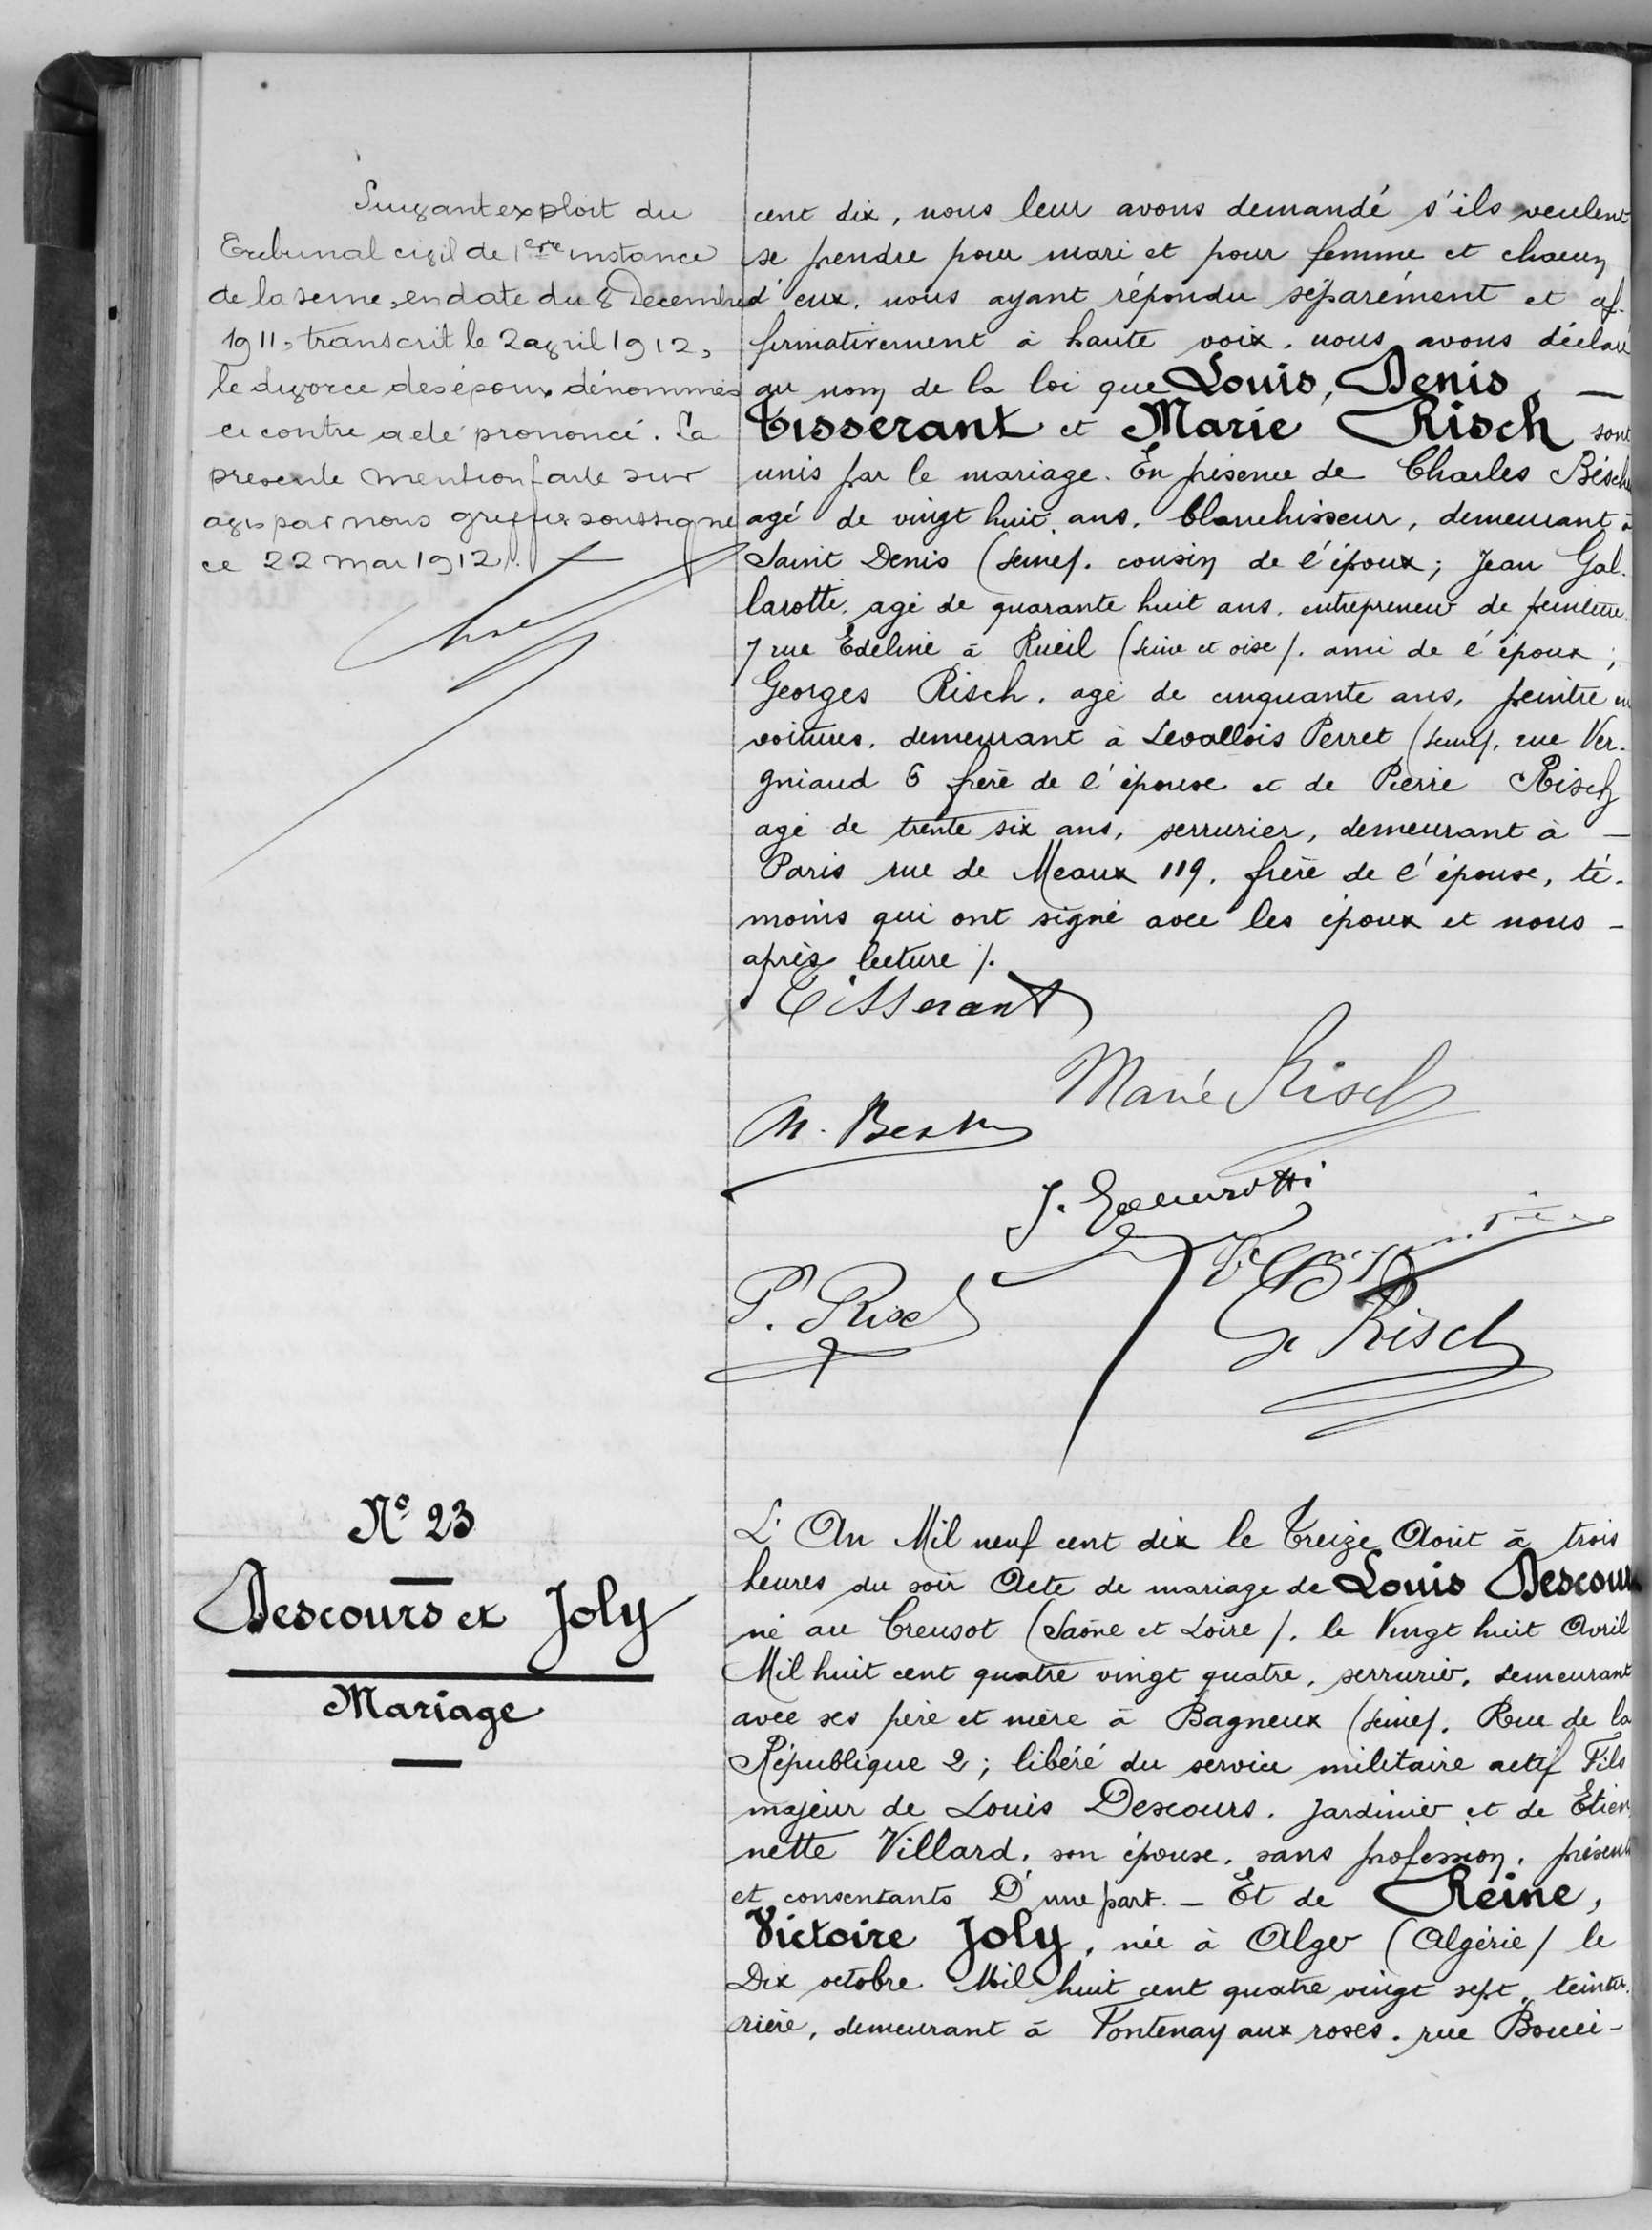cent dix, nous leur avons demandé s'ils veulent
se prendre pour mari et pour femme et chacun
d'eux. nous ayant répondu séparément et af-
firmativement à haute voix. nous avons déclaré
au nom de la loi que Louis, Denis -
Tisserant et Marie Risch sont
unis par le mariage. En présence de Charles Bésch
agé de vingt huit ans. blanchisseur, demeurant à
Saint Denis (Seine). cousin de l'époux; Jean Gal-
larotte, agé de quarante huit ans. entrepreneur de fermeture
7 rue Edeline à Rueil (Seine et Oise). ami de l'époux;
Georges Risch. agé de cinquante ans, peintre sur
voiture. demeurant à Levallois Perret (Seine). rue Ver-
gniaud 6 frère de l'épouse et de Pierre Risch
agé de trente six ans, serrurier, demeurant à -
Paris rue de Meaux 119. frère de l'épouse, té-
moins qui ont signé avec les époux et nous -
après lecture ./.
N°23
Descous et Joly
Mariage
L'An Mil neuf cent dix le Treize Aout à trois
heures, du soir Acte de mariage de Louis Descours
né au Creusot (Saône et Loire), le Vingt huit Avril
Mil huit cent quatre vingt quatre, serrurier, demeurant
avec ses père et mère à Bagneux (Seine), Rue de la
République 2; libéré du service militaire actif Fils
majeur de Louis Descours, jardinier, et de Etien
nette Villard, son épouse, sans profession, présent
et consentants D'une part. -Et de Reine,
Victoire Joly, née à Alger (Algérie) le
Dix, octobre Mil huit cent quatre vingt sept, teintu
rière, demeurant à Fontenay aux roses. rue Bouci-
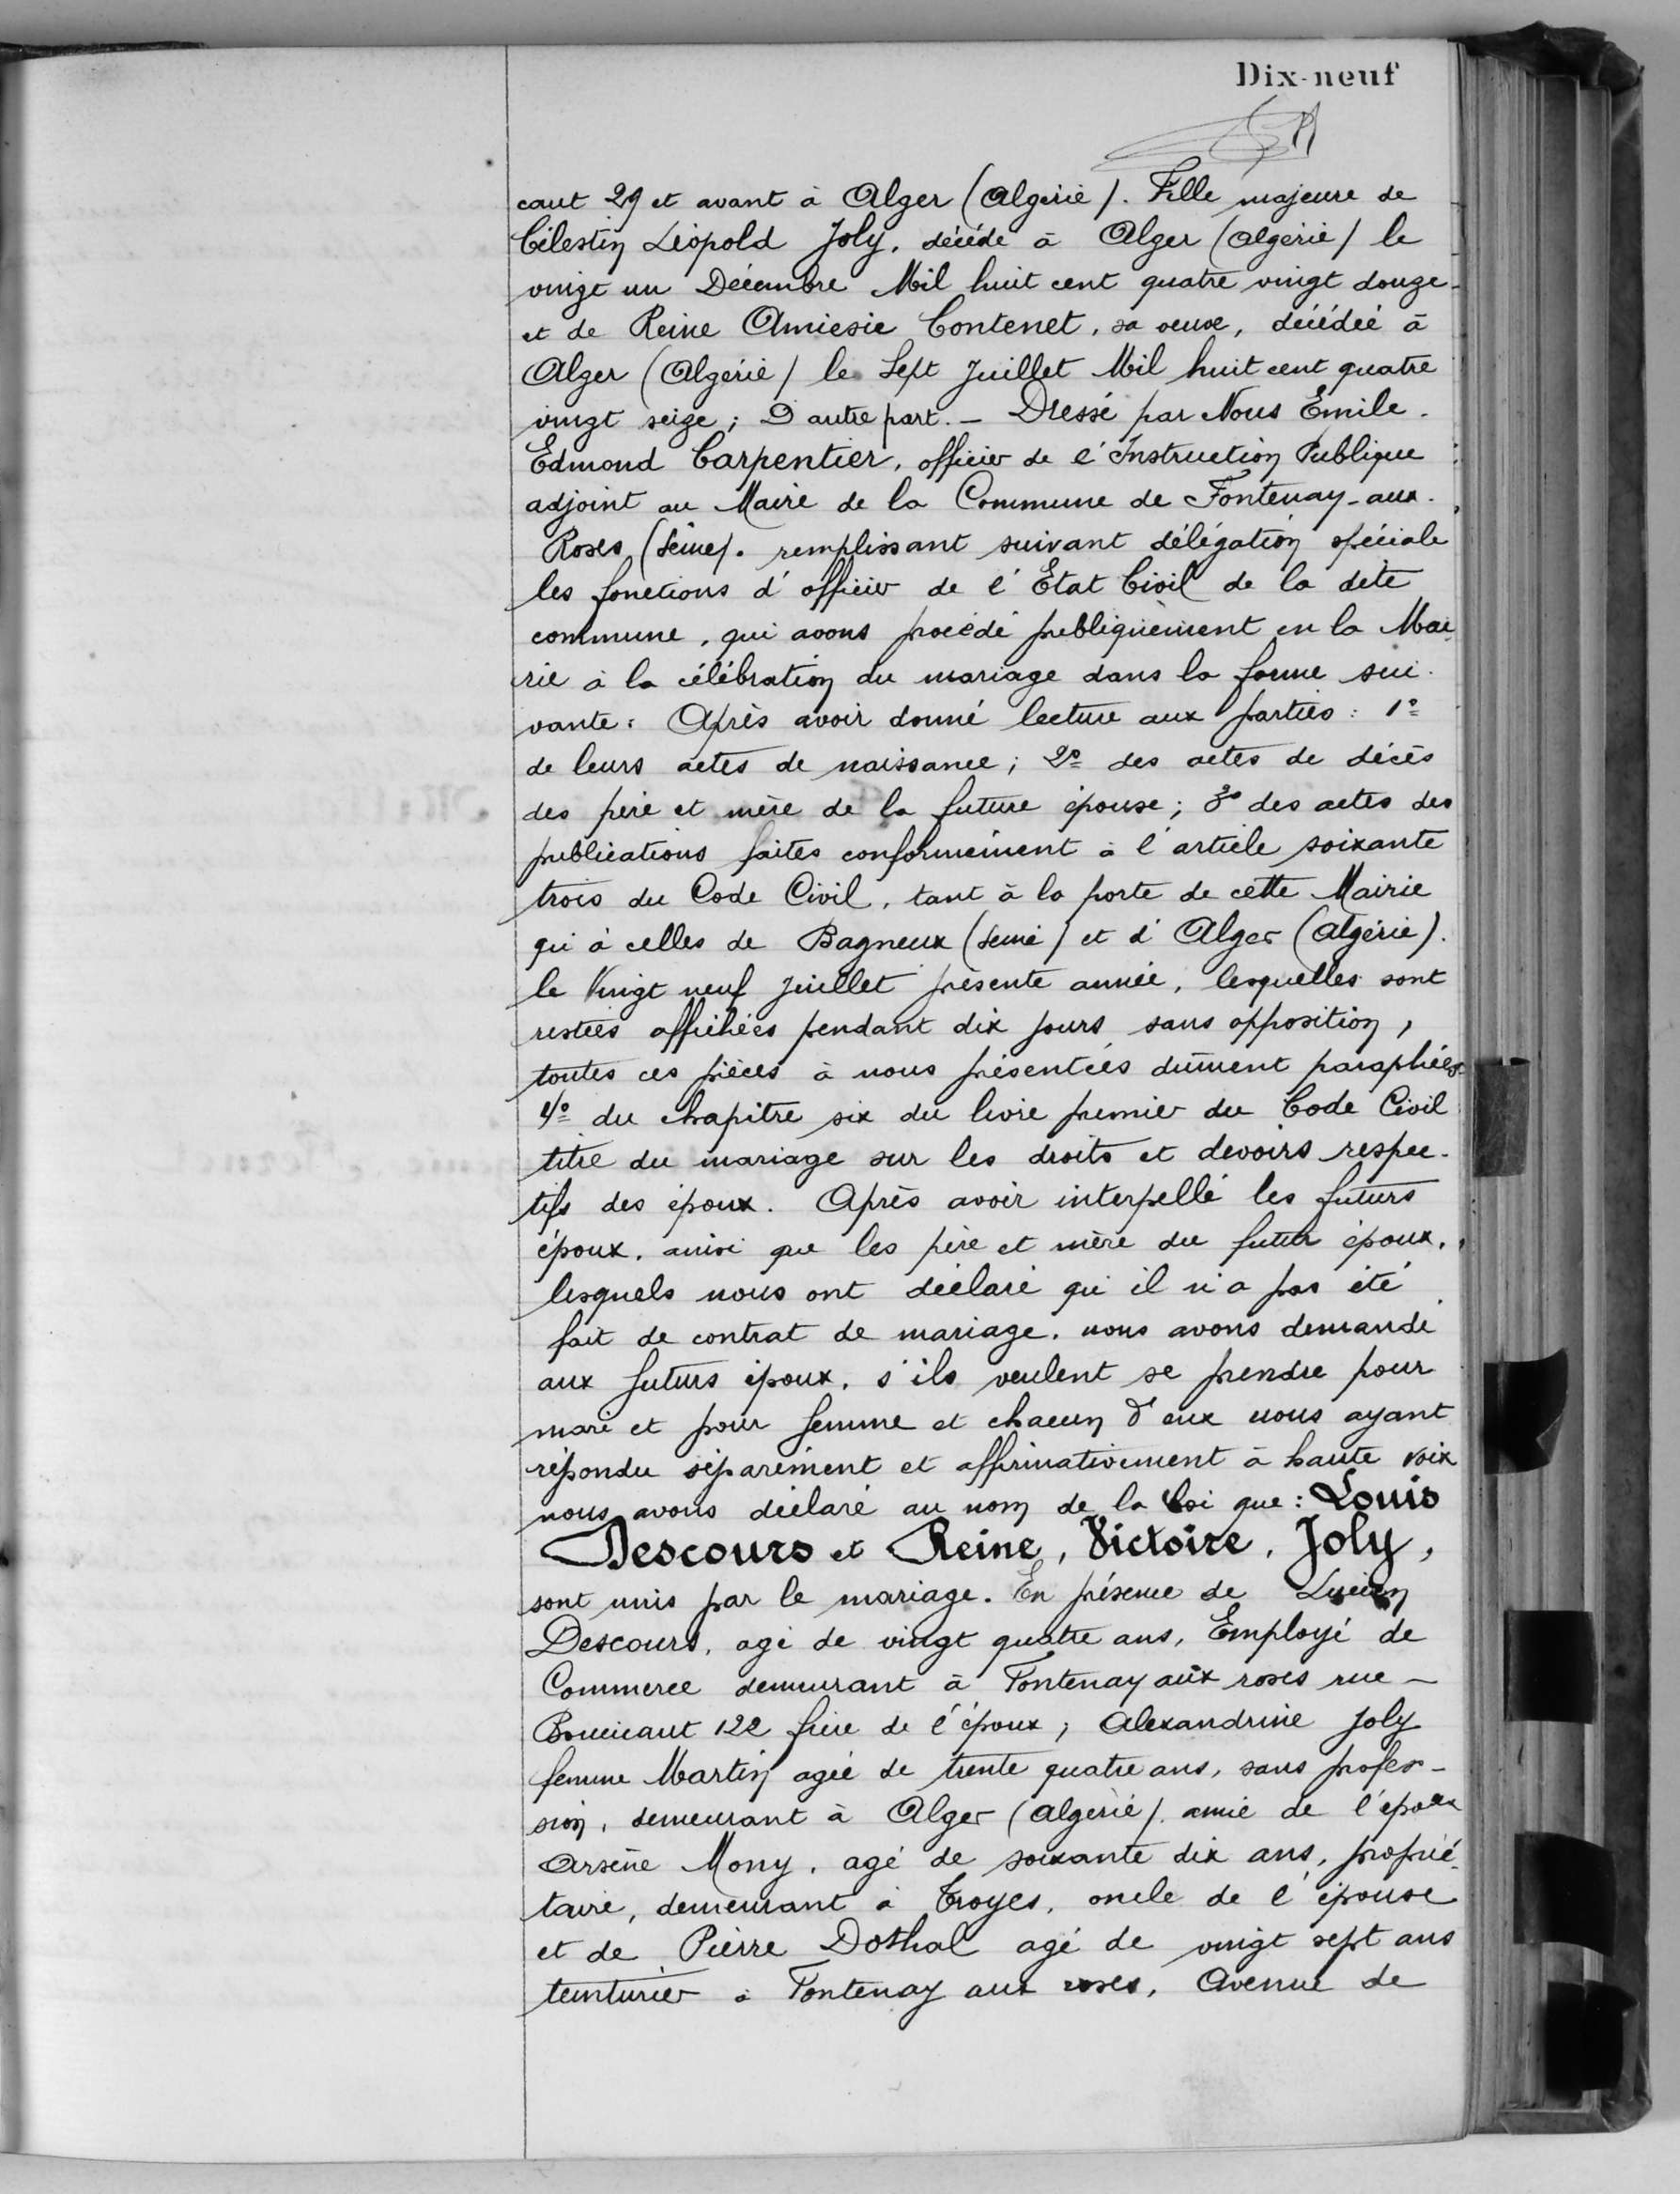caut 29 et avant à Alger (Algérie). Fille majeure de
Célestin Léopold Joly, décédé à Alger (Algérie) le
vingt un Décembre Mil huit cent quatre vingt douze-
et de Reine Amiesie Contenet, sa veuve, décédée à
Alger (Algérie) le Sept Juillet Mil huit cent quatre
vingt seize; D'autre part.-Dressé par Nous Emile-
Edmond Carpentier, officier de l'Instruction Publique
adjoint au Maire de la Commune de Fontenay-aux
Roses (Seine). remplissant suivant délégation spéciale
les fonctions d'officier de l'Etat Civil de la dite
commune, qui avons procédé publiquement en la Mai
rie à la célébration, du mariage dans la forme sui
vante. Après avoir donné lecture aux parties: 1°
de leurs actes de naissance; 2° des actes de décès
des père et mère de la future épouse; 3° des actes des
publications faites conformément à l'article soixante
trois du Code Civil, tant à la porte de cette Mairie
qui à celles de Bagneux (Seine) et d'Alger (Algérie).
le Vingt neuf juillet présente année, lesquelles sont
restées affichées pendant dix jours, sans opposition,
toutes ces pièces à nous présentées dûment paraphées
2° du chapitre six du livre premier du Code Civil
titre du mariage sur les droits et devoirs respec-
tifs des époux. Après avoir interpellé les futurs
époux. ainsi que les père et mère du futur époux,
lesquels nous ont déclaré qu'il n'a pas été
fait de contrat de mariage. nous avons demandé
aux futurs époux, s'ils veulent se prendre pour
mari et pour femme et chacun d'eux nous ayant
répondu séparément et affirmativement à haute voix
nous avons déclaré au nom de la loi que: Louis
Descours et Reine, Victoire, Joly,
sont unis par le mariage. En présence de Lucien
Descours, agé de vingt quatre ans, Employé de
Commerce demeurant à Fontenay aux roses sur-
Boucicaut 122, frére de l'époux, Alexandrine Joly
femme Martin agé de trente quatre ans, sans profes-
sion, demeurant à Ager (Algérie) amie de l'épouse
Arsène Mony, agé de soixante dix ans, proprié
taire, demeurant à Troyes, oncle de l'épouse
et de Pierre Dorthal agé de vingt sept ans
teinturier à Fontenay aux roses, Avenue de